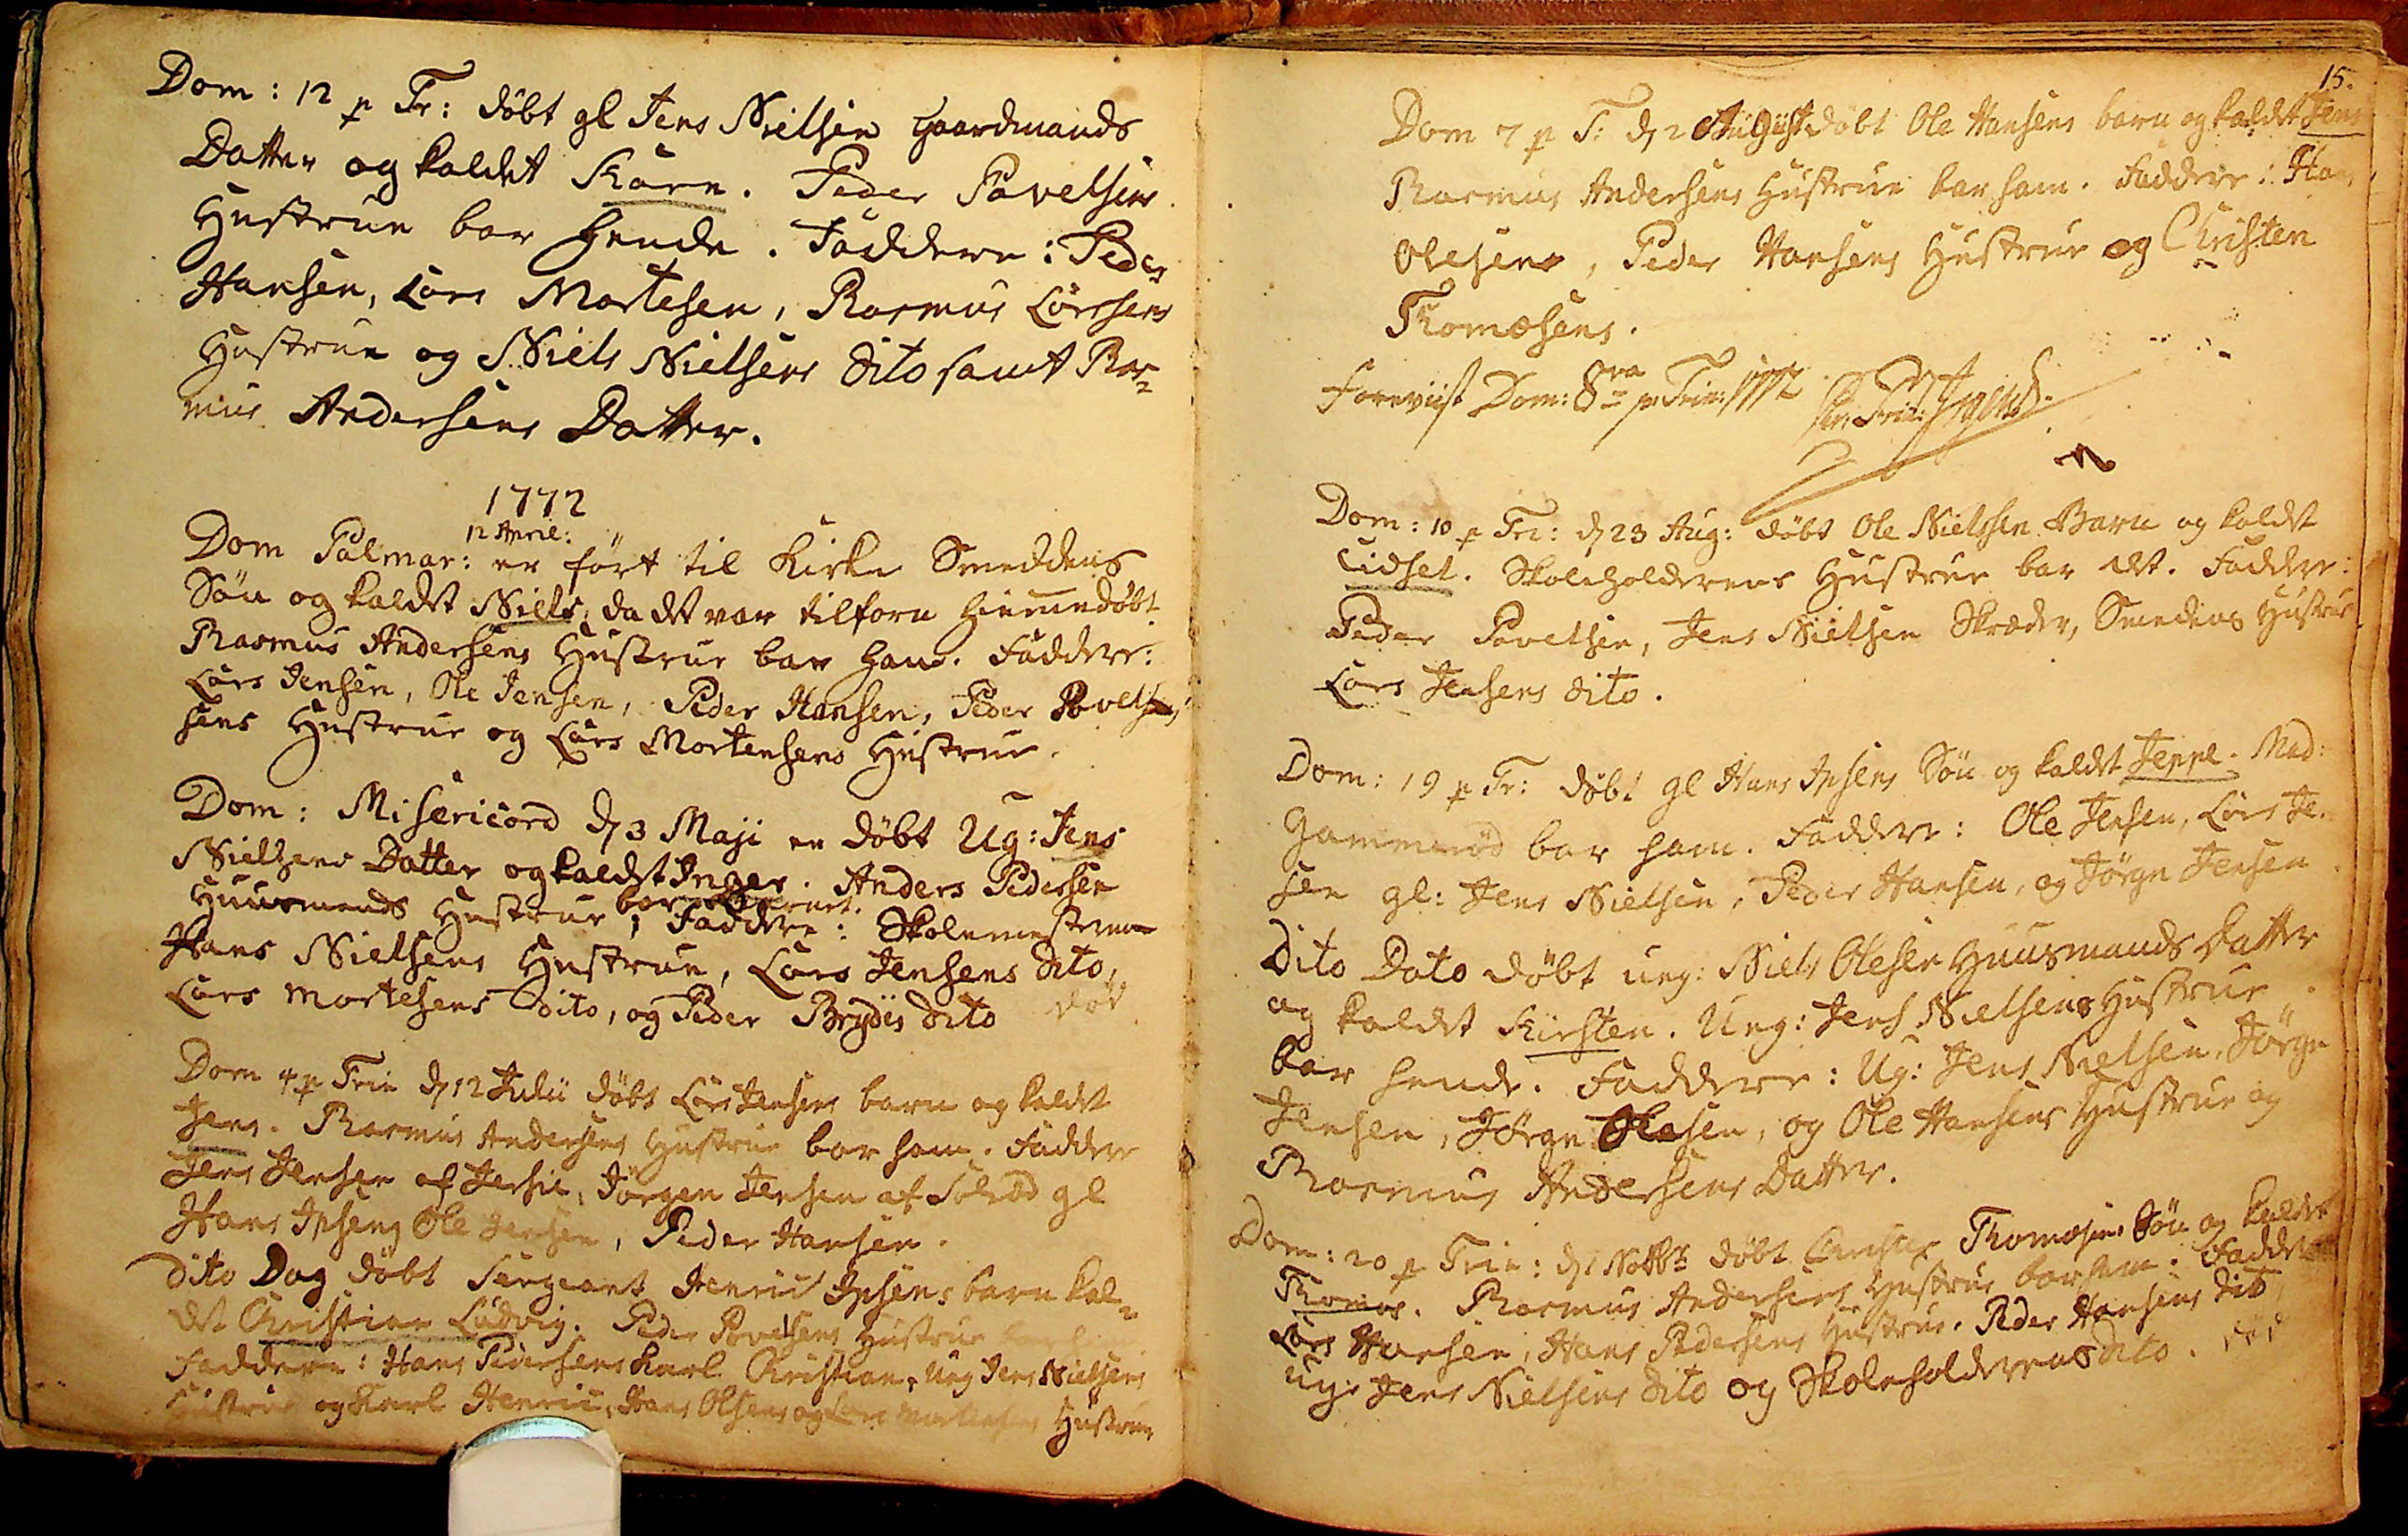Dom: 12 p Tr: døbt gl Jens Nielsen Gaardmands
Datter og kaldet Karen. Peder Povelsens
Hustrue bar hende. Faddere: Peder
Hansen, Lars Mortesen, Rasmus Larssens
Hustrue og Niels Nielsens dito samt Ras¬
mus Andersens Datter.
1772
12 April:
Dom Palmar: er ført til Kirke Smeddens
Søn og kaldet Niels. da det var tilforn hiemedøbt
Rasmus Andersens Hustrue bar ham. Faddere:
Lars Jensen, Ole Jensen, Peder Hansen, Peder Povelsen,
sens Hustrue og Lars Mortensens Hustrue.
Dom: Misericord d 3 Maji er døbt Ug: Jens
Nielsens Datter og kaldet Inger. Anders Pedersen
bar Barnet
Huusmands Hustrue , Faddere: Skolemesteren
Hans Nielsens Hustrue, Lars Jensens dito,
Lars Mortesens dito, og Peder Brydes dito
død
Dom 4 p Trin d 12 Julii døbt Lars Jensens barn og kaldt
Jens. Rasmus Andersens Hustrue bar ham. Faddere
Jens Jensen af Jersie, Jørgen Jensen af Solrød gl
Hans Ipsen, Ole Jensen, Peder Hansen.
Dito Dag døbt Sergeant Henric Ipsens barn kal¬
det Christian Ludvig. Peder povelsens Hustrue bar ham.
Faddere: Hans Pedersens Karl Christian, Ung Jens Nielsens
Hustrue og Karl Henric, Hans Olsens og Lars Mortensens Hustruer
15.
Dom 7 p Tr: d 2 August døbt Ole Hansens barn og kaldet Jens
Rasmus Andersens Hustrue bar ham. Faddere: Hans
Olesen, Peder Hansens Hustrue og Christen
Thomæsens.
Foreviist Dom: 8va p: Trin: 1772
Chr: Frid: Jrgens ./.
Dom: 10 p Tri: d 23 Aug: døbt Ole Nielssen Barn og kaldt
Cidsel. Skoleholderens Hustrue bar det. Faddere:
Peder Povelsen, Jens Nielsen Skræder, Smedens Hustrue
Lars Jensens dito.
Dom: 19 p Tr: døbt gl Hans Ipsens Søn og kaldet Jeppe. Mad:
Gammerød bar ham. Faddere: Ole Jensen, Lars Je
sen gl: Jens Nielsen, Peder Hansen, og Jørgen Jensen.
Dito Dato døbt ung: Niels Olesen Huusmands Datter
og kaldt Kirsten. Ung: Jens Nielsens Hustrue
bar hende. Faddere: Ug: Jens Nielsen, Jørgn
Jensen, Jørgn Olesen, og Ole Hansens Hustrue og
Rasmus Andersens Datter.
Dom: 20 p Trin: d 1 Novbr døbt Christen Thomæsens Søn kaldt
Thomas. Rasmus Andersens Hustrue bar ham. Faddere
Lars Hansen, Hans Pedersens Hustrue, Peder Hansens dito,
ug: Jens Nielsens dito og Skoleholderens dito.
død